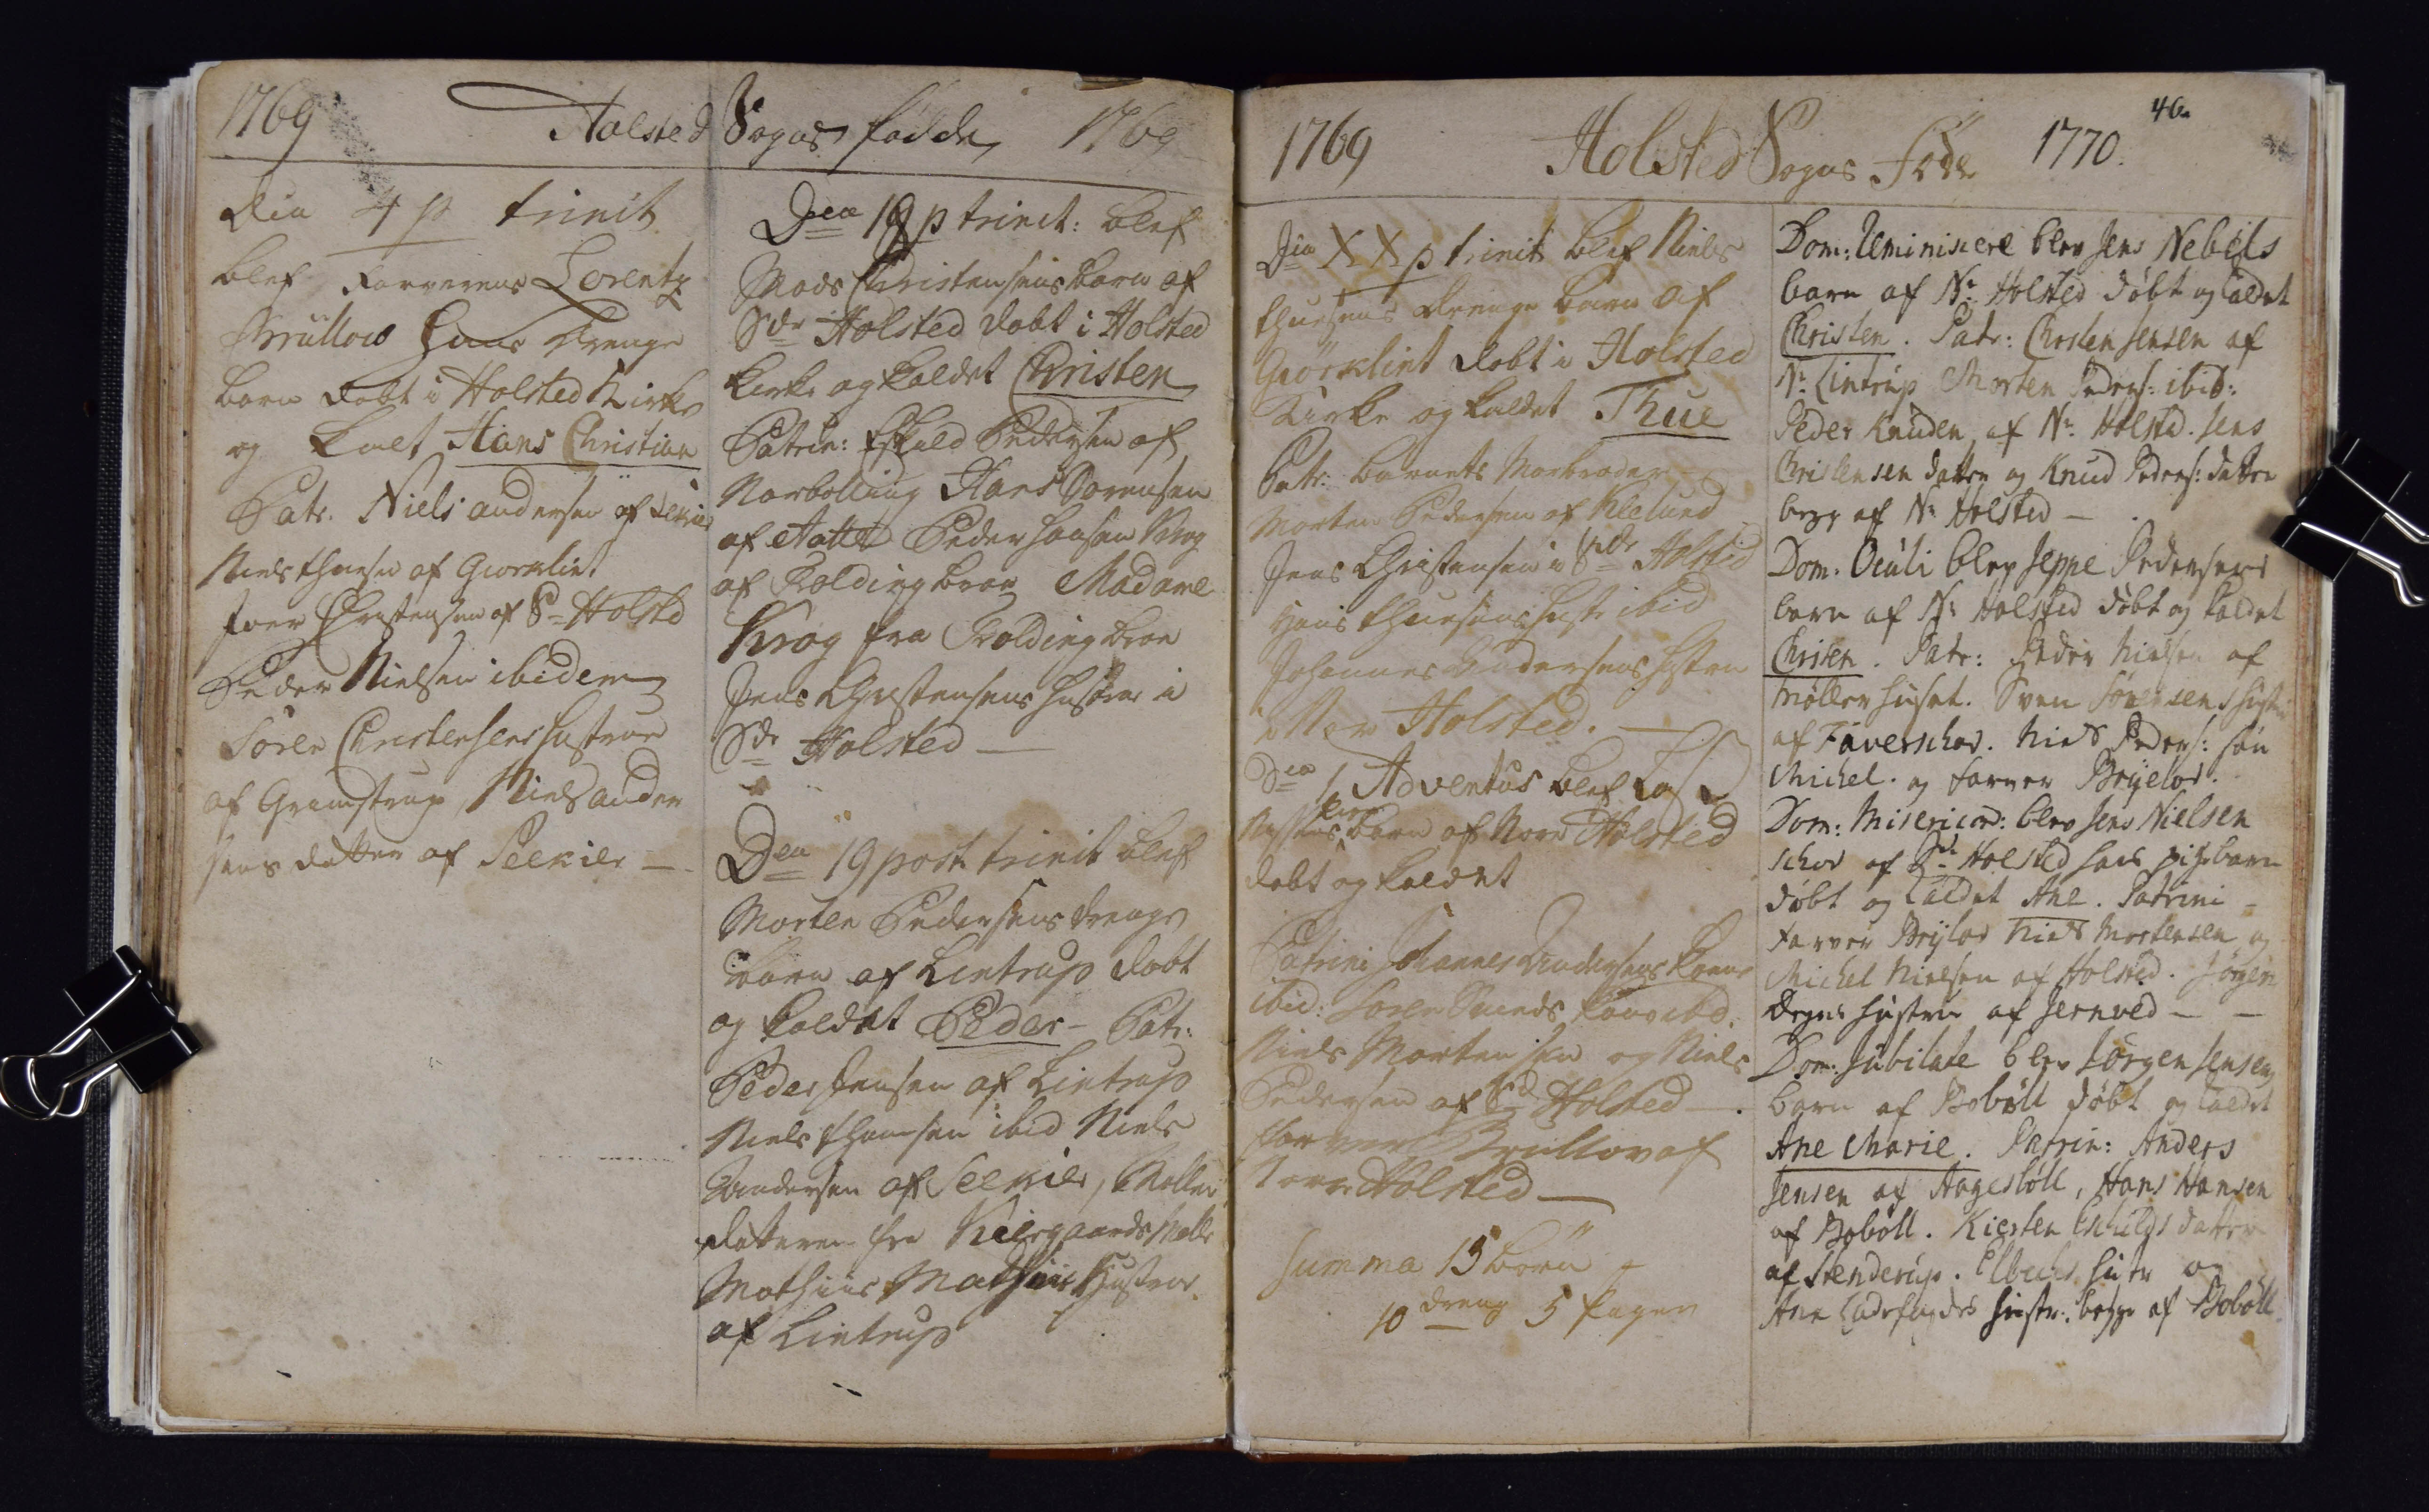1769
Holsted Sogne fødde
Dca 4 p Trinit
blef Farverens Lorentz
Brullous Hans Dreng
barn døbt i Holsted Kirke
og kalt Hans Christian
Patr. Niels Andersen af Sekier
Niels Thuesen af Giorklint
Iver Christensen af Sr Holsted
Peder Nielsen ibidem
Søren Christensens Hustrue
af Grimstrup, Niels Ander
sens datter af Sekier -
1769.
Dca 18 p Trinit: blef
Mads Christensens barn af
Sdr Holsted dobt i Holsted
Kirke og kaldet Christen
Patrin: Eskild Pedersen af
Norbolling Hans Sørensen
af Aatte Peder Hansen Krog
af Kolding broe Madame
Krog fra Kolding broe
Jens Christensens Hustru i
Sdr Holsted
Dca 19 post Trinit blev
Morten Pedersens Drenge
Barn af Lintrup dobt
og kaldet Peder Patr:
Peder Jensen af Lintrup
Niels Hansen ibid Niels
Andersen af Seekier, Moller
datteren fra Kiergaards Mølle
Mathiis Mathiis Hustrue
af Lintrup
1769
Holsted Søns Føde
Dca XX p Trinit bleff Niels
Thuesens Drenge barn af
Giørklint døbt i Holsted.
Kirke og kaldet Thue
Patr: barnets Morbroder
Morten Pedersen af Klelund
Jens Christensen i Sdr Holsted
Hans Thuesens Hustr ibid
Johannes Andersens Hustru
i Nor Holsted.
Dca Adventus blef Lass
Pige
Nissens barn af Nor Holsted
døbt og kaldet
Patrini Johannes Andersens Koene
ibid: Soren Smeds Kone ibd:
Niels Mortensen og Niels
Pedersen af Sdr Holsted
Farver Brulov af
Norre Holsted -
Samma 15 Børn
10 Dreng 5 Piger
46.
1770
Dom: Reminiscere blev Jens Nebels
Barn af Nr Holsted døbt og kaldet
Christen. Patr: Christen Jensen af
Nr Lintrup Morten Peders: ibid:
Peder Knudsen af Nr Holsted. Jens
Christensen Datter og Knud Peders: datter
begge af Nr Holsted -
Dom: Oculi blev Jeppe Pedersens
barn af Nr Holsted døbt og kaldet
Christen Patr: Peder Nielsen af
Møller Huset. Sven Sørnsens Hustru
af Faverschov. Niels Peders: søn
Michel. og farver Brelov.
Dom: Misericord: blev Jens Nielsen
Schov af Sdr Holsted hans pigebarn
døbt og kaldet Ane. Patrini
Farver Brylov Niels Mortensen og
Michel Nielsen af Holsted. Jørgen
Degns Hustrue af Jernved -
Dom: Jubilate blev Jørgen Jensens
Barn af Bobøll døbt og kaldet
Ane Marie. Patrin: Anders
Jensen af Aagesbøll, Hans Hansen
af Bobøll. Kiersten Eschilds datter
af Stenderup. Elbechs hustr og
Ane Ladefogedes Hustr: begge af Bobøll
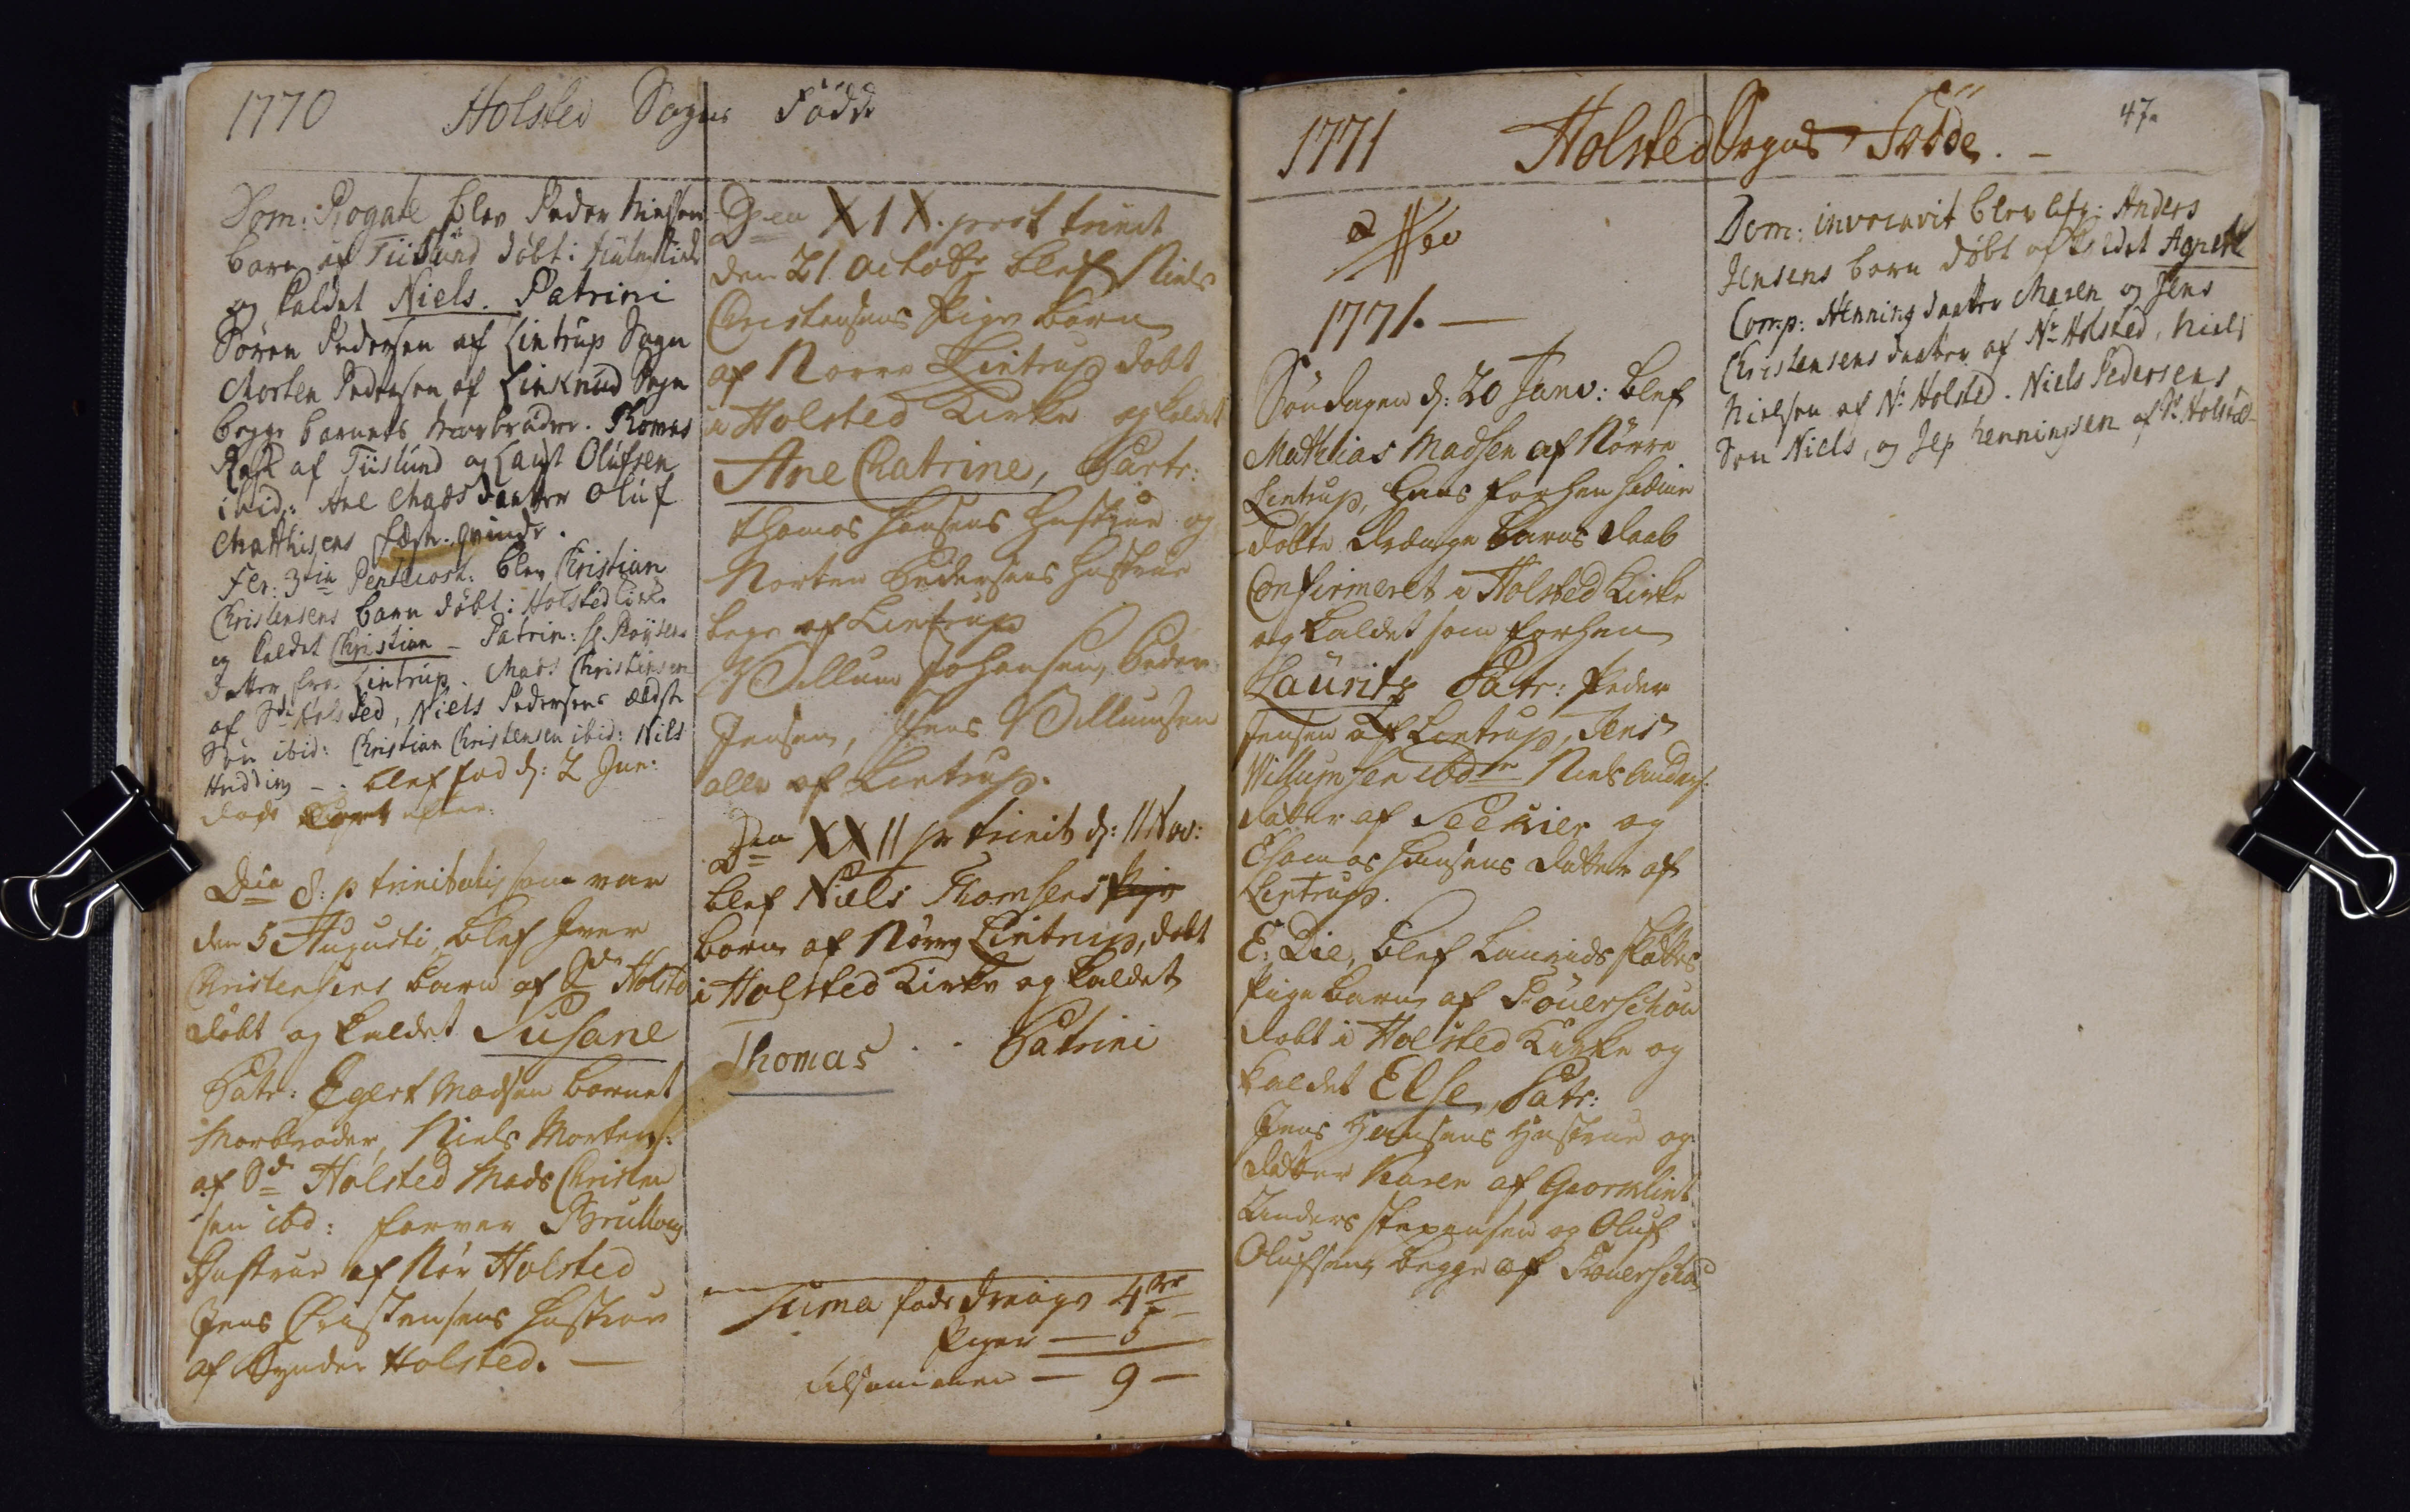Holsted Sogns Fødde
1770
Dom: Rogate blev Peder Nielsen
Barn af Tiislund døbt i Feuling Kirke
og kaldet Niels Patrini
Søren Pedersen af Lintrup Sogn
Morten Pedersen af Linknud Sogn
begge barnets Morbrødre. Thomas
Rask af Tiislund og Laust Olufsen
ibid: Ane Madsdaatter Oluf
Matthisens Fæste:Qvinde.
Fer: 3tia Pentecost: blev Christian
Christensens barn døbt Holsted Kirke
og kaldet Christian - Patrin: Hl: Ploy##
datter fra Lintrup. Mads Christensen
af Sdr Holsted, Niels Pedersens ældste
Søn ibid: Christian Christensen ibid: Niels
Hvidding - - blef fod d: 2 Jun:
dode kort efter.
Dca 8: p Trinitatis som var
Den 5 Augusti blef Iver
Christensens barn af Sdr Holsted
døbt og kaldet Susane
Patr: Egert Madsen barnet
Morbroder, Niels Mortens:
af Sdr Holsted, Mads Christen
sen ibd: farver Brulloeg
Hustrue af Nør Holsted
Jens Christensens Hustru
af Synder Holsted.
Dca 21 post trinit
den 21. Octobr bleff Niels
Christensens Pige barn.
af Norre Lintrup dobt
i Holsted Kirke og kaldet
Anne Chatrine, Partr:
Thomas Hansens Hustrue og
Morten Pedersens Hustrue
bege af Lintrup
Villum Johansen, Peder
Jensen, Jens Ollumsen
alle af Lintrup
Dca XXII p Trinit d: 11 Nov:
blef Niels Thomsens Pige
barn af Nørre Lintrup, døbt
i Holsted Kirke og kaldet
Thomas.. Patrini
Suma fode Drenge 4##
Piger - 5 
tilsammen - 9 -
Holsted Sogns Fødde
1771
1771.
Søndagen d: 20 Janv: blef
Mathias Madsen af Nørre
Lintrup, Hans forhen hiæme
døbte dedage barns daab
Confirmeret i Holsted Kirke
og kaldet som forhen
Lauritz Patr: Peder
Jensen af Lintrup, Jens
Willumsen ibdm Niels Anders:
datter af Seekier og
Thomas Hansens Datter af
Lintrup
E: Die blef Laurids skattes
Pige barn af Fouerschou
døbt i Hoelsted Kirke og
kaldet Else Patr:
Jens Hansens Hustrue og
Datter Karen af Giorklint
Anders Stepensen og Oluf
Olufsen, begge af Fouerschou
47
Dom: Invocavit blev afg: Anders
Jensens barn døbt og kaldet Agner
Comp: Henning datter Maren og Jens
Christensens daatter af N: Holsted, Niels
Nielsen af N: Holsted. Niels Pedersens
Søn Niels, og Jep henningsens af Sr Holsted